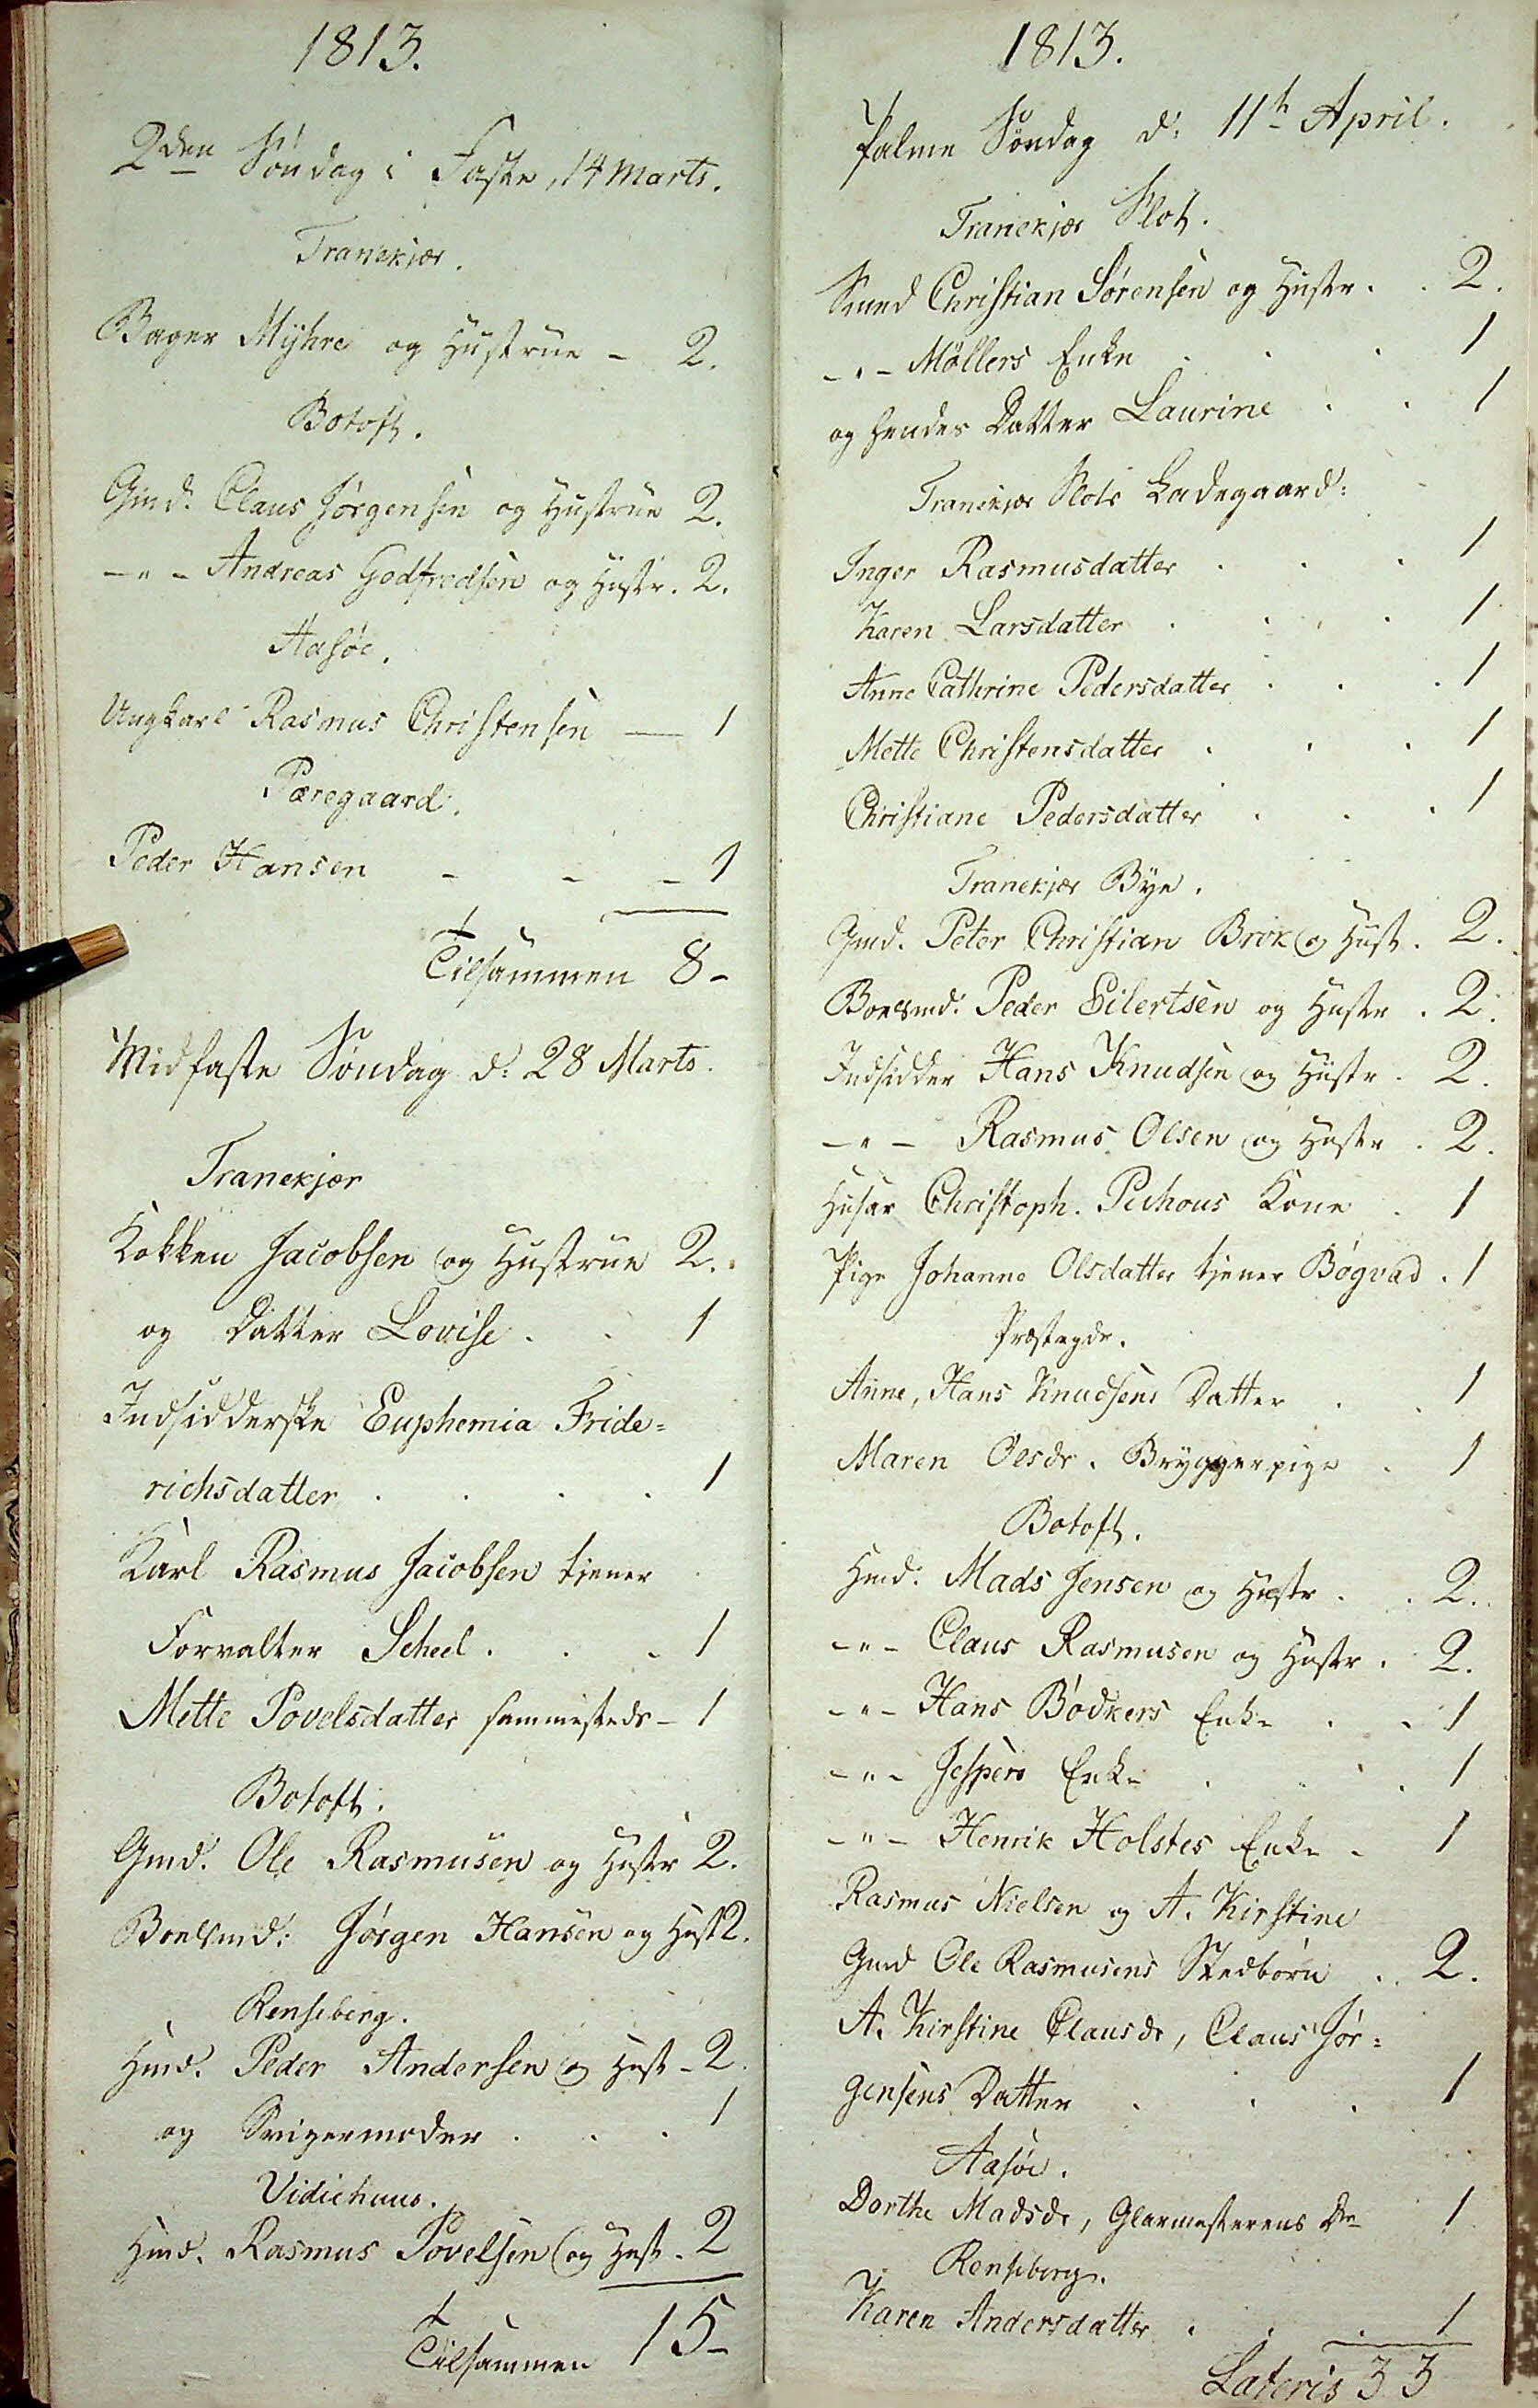1813.
2den Søndag i Faste, 14 Marts.
Tranekjær.
Bager Myhre og Hustrue - 2.
Botoft.
Gmd. Claus Jørgensen og Hustrue 2.
-"- Andreas Godfredsen og Hustr . 2.
Aasøe.
Ungkarl Rasmus Christensen - 1
Pæregaard.
Peder Hansen - - - 1
Tilsammen 8-
Midfaste Søndag d: 28 Marts.
Tranekjær
Kokken Jacobsen og Hustrue 2..
og Datter Lovise . . 1
Indsidderske Euphemia Frider¬
richsdatter . . . . 1
Karl Rasmus Jacobsens tjener
Forvalter Scheel . . . 1
Mette Povelsdatter sammesteds - 1
Botoft.
Gmd: Ole Rasmusen og Hustr 2.
Boelsmd. Jørgen Hansen og Hust 2.
Renseberg.
Hmd. Peder Andersen og Hust - 2.
og Svigermoder . . . 1
Vidiehuus.
Hmd. Rasmus Povelsen og Hust . 2
Tilsamme 15-
1813.
Palme Søndag d. 11te April.
Tranekjær Slot
Smed Christian Sørensen og Hustr . . 2.
-"- Møllers Enke . . . 1
og hendes Datter Laurine . . 1
Tranekjær Slots Ladegaard:
Inger Rasmusdatter . . . 1
Karen Larsdatter . . . . 1
Anne Cathrine Pedersdatter . . . 1
Mette Christensdatter . . . 1
Christiane Pedersdatter . . . 1
Tranekjær Bye.
Gmd. Peter Christian Brok og Hust . 2.
Boelsmd. Peder Eilertsen og Hustr . 2.
Indsidder Hans Knudsen og Hustr . 2.
-"- Rasmus Olsen og Hustr: 2.
Husar Christoph. Pichous Kone . 1
Pige Johanne Olsdatter tjener Bøgvad . 1
Præstegdr.
Anne, Hans Knudsens Datter . .1
Maren Olsdr. Bryggerpige . 1
Botoft.
Hmd. Mads Jensen og Hustr . . 2..
-"- Claus Rasmusen og Hustr . 2.
-"- Hans Boders Enke -
-"- Jespers Enke . . . . 1
-"- Henrik Holstes Enke . 1
Rasmus Nielsen og A. Kirstine
Gmd Ole Rasmusens Stedbørn . 2.
A. Kirstine Clausdr, Claus Jør¬
gensens Datter . . . 1
Aasøe.
Dorthe Madsdr, Glarmesterens Dr 1
Renseberg.
Karen Andersdatter . . . 1
Lateris 33
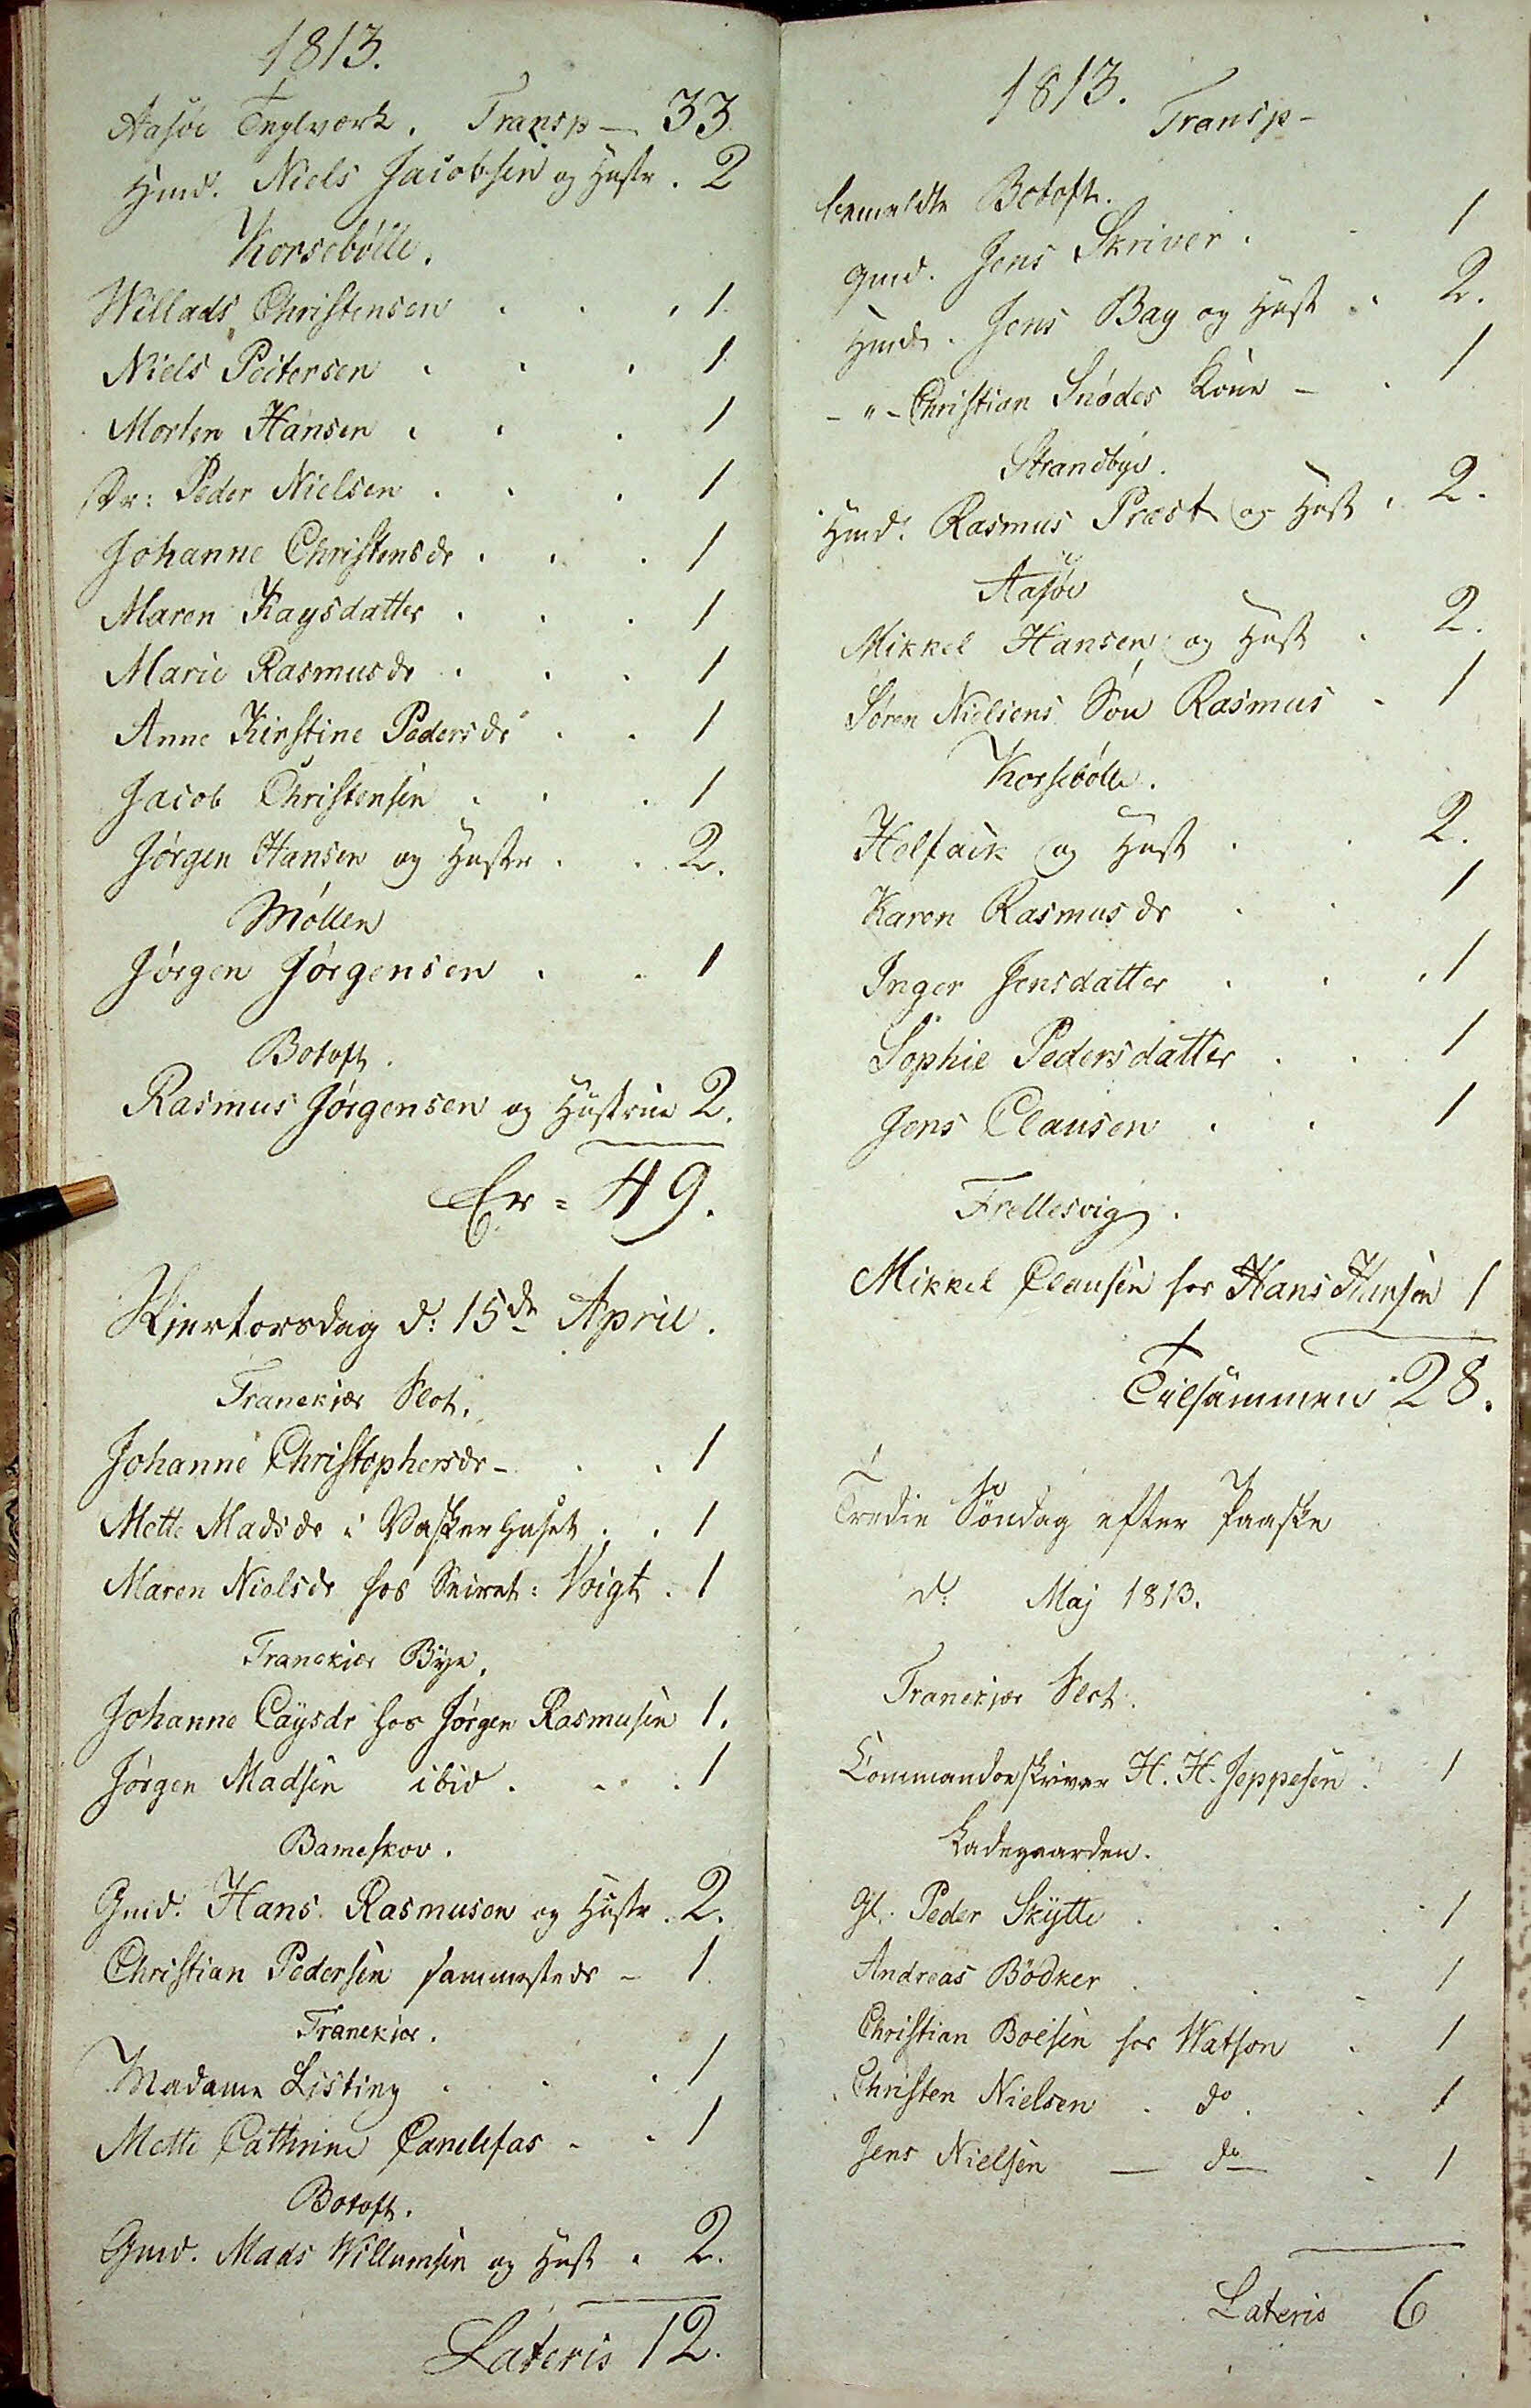1813.
Aasøe Teglværk. Transp - 33.
Hmd. Niels Jacobsen og Hustr . 2
Korsebølle.
Willads Christensen . . . 1.
Niels Peitersen . . . 1
Morten Hansen . . . 1
Dr: Peder Nielsen. . . 1
Johanne Christensdr . . . 1
Maren Kaysdatter . . . 1
Marie Rasmusdr . . . 1
Anne Kirstine Pedersdr . . 1
Jacob Christensen . . . 1
Jørgen Hansen og Hustr . . 2.
Møllen
Jørgen Jørgensøn . . 1
Botoft
Rasmus Jørgensen og Hustrue 2.
Er= 49
Skiertorsdag d: 15de April.
Tranekjær Slot.
Johanne Christophersdr . . . 1
Mette Madsdr i Vaskenhuset . . 1
Maren Nielsdr hos Secret: Voigt . 1
Tranekjær Bye.
Johanne Caysdr hos Jørgen Rasmusen 1.
Jørgen Madsen ibid . . . 1
Bameskov.
Gmd. Hans Rasmusen og Hustr . 2.
Christian Pedersen sammesteds - 1.
Tranekjær.
Madame Listing . . . . 1
Mette Cathrine Canelefas . . 1
Botoft.
Gmd. Mads Willumsen og Hust . 2.
Lateris 12.
1813.
Transp-
bemeldte Botoft.
Gmd. Jens Skriver . . 1
Hmd. Jens Bay og Hust . . : 2.
-"- Christian Snødes Kone - . 1.
Strandbye.
Hmd. Rasmus Præst og Hust . 2.
Aasøe
Mikkel Hansens og Hust . 2.
Søren Nielsens Søn Rasmus . 1
Korsebølle.
Helfack og Hust . . 2.
Karen Rasmusdr . . 1
Inger Jensdatter . . 1
Sophie Pedersdatter . . . 1
Jens Clausen . . 1
Frellesvig
Mikkel Clausen hos Hans Hansen 1
Tilsammen 28.
Tredie Søndag efter Paaske
d: Maj 1813.
Tranekjær Slot.
Commandoeskriver H. H. Jeppesen . 1
Ladegaarden.
Gl. Peder Skytte . . . . 1
Andreas Bødker . . . 1
Christian Boesen hos Watson . 1
Christen Nielsen . do . . . 1
Jens Nielsen - do - . 1
Lateris 6